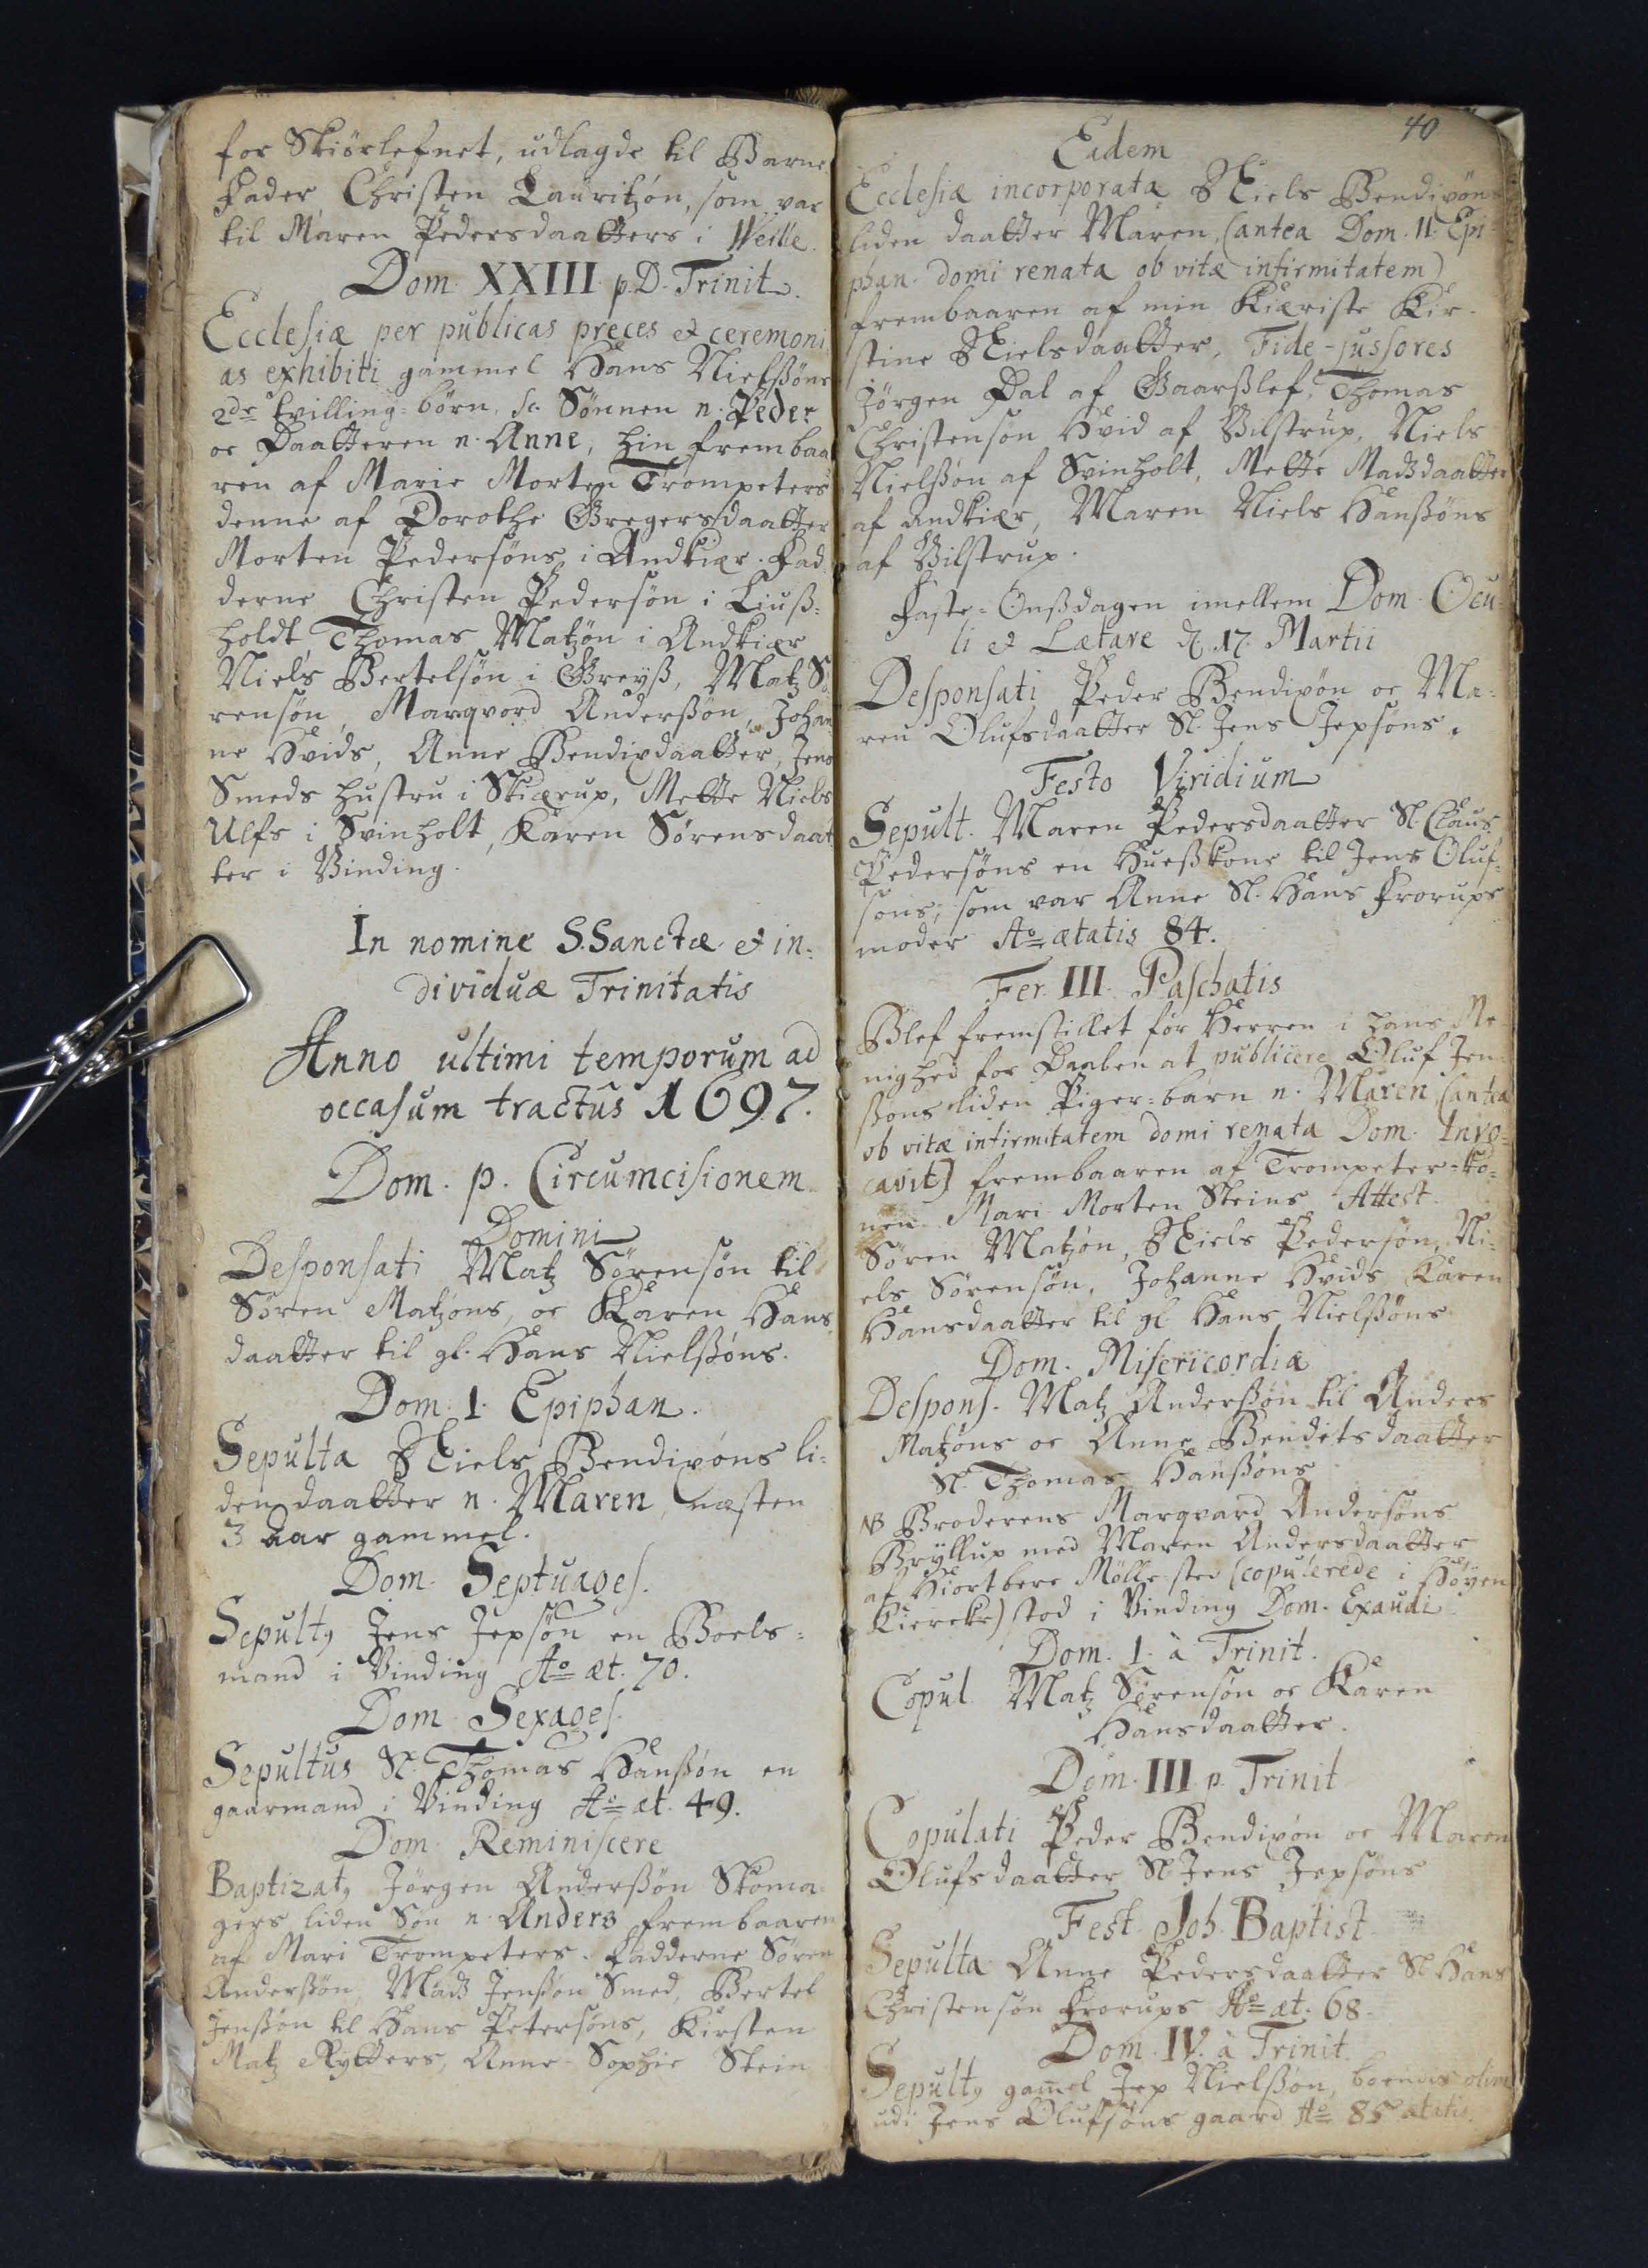for Skiørlefnet, udlagde til Barne
Fader, Christen Lauritzøn, som var
til Maren Pedersdaatters i Weille
Dom. XXIII p. D. Trinit
Ecclesiæ per publicas preces et ceremoni 
as exhibiti gammel Hans Nielsøns
2de tvilling=børn, sc. Sønnen n. Peder
oc Daatteren n. Anne, hin frembaa¬
ren af Marie Morten Trompeters
denne af Dorothe Gregersdaatter
Morten Pedersøns i Andkiær. Fad¬
derne. Christen Pedersøn i Liuss¬
holdt Thomas Matzøn i Andkiær
Niels Bertelsøn i Greyss, Matz Sø¬
rensøn, Marqvard Anderssøn, Johan¬
ne Hvids, Anne Bendixdaatter, Jens
Smeds hustru i Skiærup, Mette Niels
Ulfs i Svinholt, Karen Sørensdaat¬
ter i Vinding
In nomine S.Sanctæ et in¬
dividuæ Trinitatis
Anno ultimi temporum ad
occasum tractus 1697
Dom. p. Circumcisionem
Domini
Desponsati Matz Sørensøn til
Søren Matzøns, oc Karen Hans
daatter til gl. Hans Nielsøns.
Dom. I. Epiphan.
Sepulta Niels Bendixøns li¬
den daatter n. Maren, næsten
3 Aar gammel.
Dom. Septuages.
Sepultq Jens Jepsøn en Boels¬
mand i Vinding Ao æt. 70
Dom. Sexages. 
Sepultus Sl. Thomas Hanssøn en
gaarmand i Vinding Ao æt. 49.
Dom. Reminiscere
Baptizatq Jørgen Anderssøn Skoma¬
gers liden Søn n. Anders frembaaren
af Mari Trompeters. Fadderne Søren
Anderssøn, Madz Jenssøn Smed., Bertel
Jenssøn til Hans Petersøns, Kirsten
Matz Rytters, Anne - Sophie Stein
40
Eodem
Ecclesiæ incorporata Niels Bendixøns
liden daatter Maren (antea Dom. II Epi
phan. domi renata ob vitæ infirmitatem)
frembaaren af min Kiæriste Kir¬
stine Nielsdaatter, Fide-jussores
Jørgen Dal af Gaarsslef, Thomas
Christensøn Hvid af Vilstrup, Niels
Nielssøn af Svinholt, Mette Madzdaatter
af Andkiær, Maren Niels Hanssøns
af Vilstrup
Faste = Onsdagen imellem Dom. Ocu¬
li et Lætare d. 17 Martii
Desponsati Peder Bendixøn oc Ma¬
ren Olufsdaatter Sl. Jens Jepsøns.
Festo Viridium
Sepult. Maren Pedersdaatter Sl. Claus
Pedersøns en Huesskone til Jens Oluf¬
søns, som var Anne Sl. Hans Frorups
moder Ao ætatis 84.
Fer. III Paschatis
Blef fremstillet for Herren i Hans Me¬
nighed for Daaben at publicere Oluf Jen¬
ssøns liden Pige=barn n. Maren, (antea
ob vitæ intirmitatem domi renata Dom. Invo¬
cavit) frembaaren af Trompetere=ko¬
nen Mari Morten Steins Attest
Søren Matzøn, Niels Pedersøn, Ni¬
els Sørensøn, Johanne Hvids Karen
Hansdaatter til gl. Hans Nielsøns
Dom. Misericordiæ
Despons. Matz Anderssøn til Anders
Matzøns oc Anne Benditsdaatter
Sl. Thomas Hanssøns
NB. Broderens Marqvard Andersøns
Bryllup med Maren Andersdaatter 
af Hiortbere Mølle=sted (copulerede i Høyen
Kiercke) stod i Vinding Dom. Exaudi
Dom. I. a Trinit
Copul. Matz Sørensøn oc Karen
Hansdaatter.
Dom. III p. Trinit
Copulati Peder Bendixøn oc Maren
Olufsdaatter Sl. Jens Jepsøns
Fest. Joh. Baptist
Sepulta Anne Pedersdaatter Sl. Hans
Christensøn Frorups Ao æt. 68
Dom. IV. a Trinit.
Sepultq gamle Jep Nielssøn, boendis
udi Jens Olufsøns gaard Ao 85 ætatis.
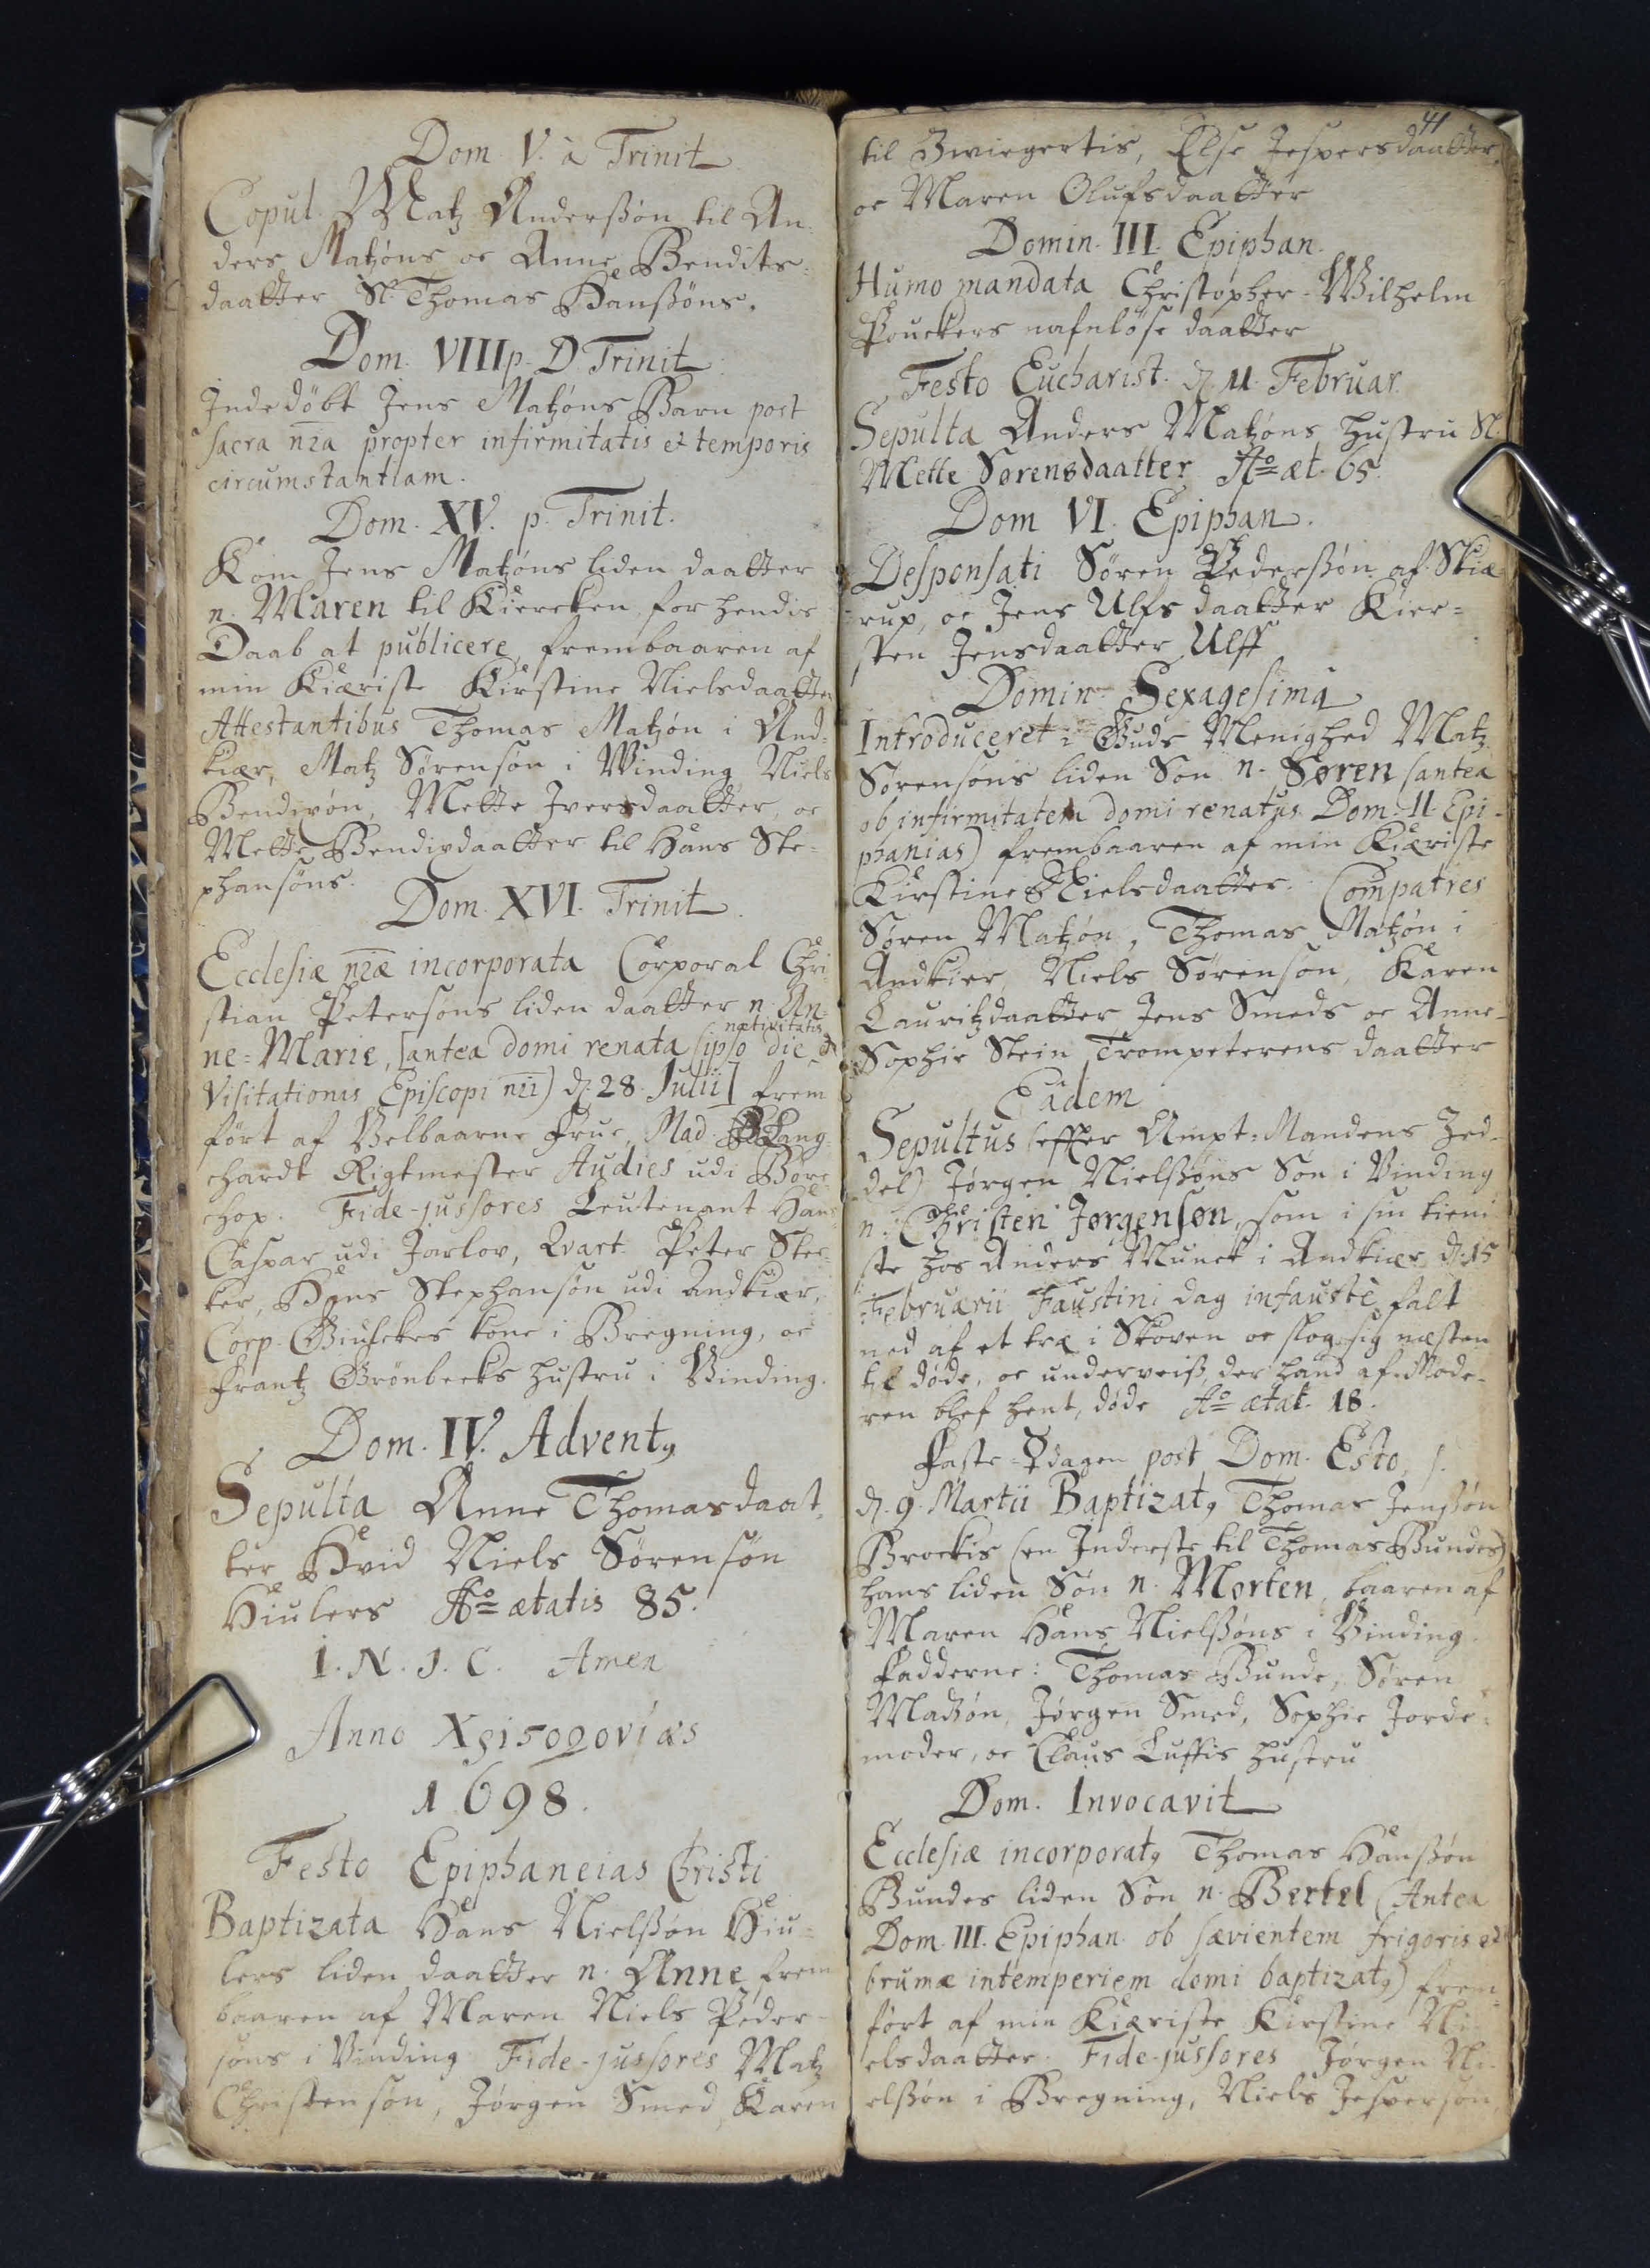Dom. V. a Trinit
Copul. Matz Anderssøn til An¬
ders Matzøns oc Anne Bendits¬
daatter Sl. Thomas Hanssøns.
Dom. VIII p. D. Trinit
Indedøbt Jens Matzøns Barn post
sacra nza propter infirmitatis et temporis
circumstantiam
Dom. XV. p. Trinit.
Kom Jens Matzøns liden daatter
n. Maren til Kiercken for hendis
Daab at publicere, frembaaren af
min Kiæriste Kirstine Nielsdaatter
Attestantibus Thomas Matzøn i And¬
kiær, Matz Sørensøn i Winding, Niels
Bendixøn, Mette Iversdaatter, oc
Mette Bendixdaatter til Hans Ste¬
phansøns.
Dom. XVI. Trinit
Ecdesiæ niæ incorporata Corporal Chri¬
stian Petersøns liden daatter n. An¬
nativitatis
ne=Marie, [antea domi renata (ipso die etc
Visitationis Episcop nii) d. 28 Julii] frem
ført af Velbaarne Frue, Mad. ## Lang¬
hardt Rigtmester Audies udi
chop. Fide-jussores. Leutenant Hans
Caspar udi Jarlov, Qvart. Peter Stec¬
ker, Hans Stephansøn udi Andkiær,
Corp. Giulckes Kone i Breining, oc
Frantz Grønbecks hustru i Vinding
Dom. IV. Adventq
Sepulta Anne Thomasdaat¬
ter Hvid Niels Sørensøn
Hiulers Ao ætatis 85
I. N. J. C. Amen
Anno X##ovias
1698
Festo Epiphaneias Christi
Baptizata Hans Nielssøn Hiu¬
lers liden daatter n. Anne, frem
baaren af Maren Niels Peder¬
søns i Vinding Fide-jussores Matz
Christensøn, Jørgen Smed. Karen
41
til Zevirgertis, Else Jespersdaatter
oc Maren Olufsdaatter
Domin. III Epiphan.
Humo mandata Christopher - Wilhelm
Pouckers nafnløse daatter
Festo Eucharist. d. 11. Februar.
Sepulta Anders Matzøns hustru Sl.
Mette Sørensdaatter Ao æt. 65.
Dom. VI. Epiphan
Desponsati Søren Pederssøn af Skiæ¬
rup, oc Jens Ulfs daatter Kier¬
sten Jensdaatter Ulff
Domin Sexagesima
Introduceret i Guds Menighed Matz
Sørensøns liden Søn n. Søren (antea
ob infirmitatem domi renatus Dom. II Epi¬
phanias) frembaaren af min Kiæriste
Kirstine Nielsdaatter. Compatres
Søren Matzøn, Thomas Matzøn i
Andkiær, Niels Sørensøn, Karen
Lauritzdaatter Jens Smeds oc Anne
Sophie Stein Trompeterens daatter.
Eadem
Sepultus (effter Ampt: Mandens Zed
del) Jørgen Nielssøns Søn i Vinding
n. Christen Jørgensøn, som i sin tieni
ste hos Anders Munck i Andkiær d. 15
Februarii Faustini dag infausté falt
ned af et træ i Skoven oc slog sig næsten 
til døde, oc underveiss, der hand af Mode¬
ren blef hent, døde Ao ætat. 18
Faste ☿ dagen post Dom. Esto,
d. 9 Martii. Baptizatq Thomas Jenssøn
Brockis (en Indserste til Thomas Bundes)
hans liden Søn n. Morten, baaren af
Maren Hans Nielssøns i Vinding.
Fadderne: Thomas Bunde, Søren
Madzøn, Jørgen Smed, Sophie Jorde¬
moder, oc Claus Luffis hustru
Dom. Invocavit
Ecclesiæ incorporat Thomas Hanssøn
Bundes liden Søn n. Bertel (Antea
Dom. III. Epiphan. ob sævientem frigoris et
brumæ intemperiem domi baptizat) frem¬
ført af min Kiæriste Kirstine Ni
elsdaatter. Fide-jussores Jørgen Ni¬
elssøn i Breining, Niels Jespersøn